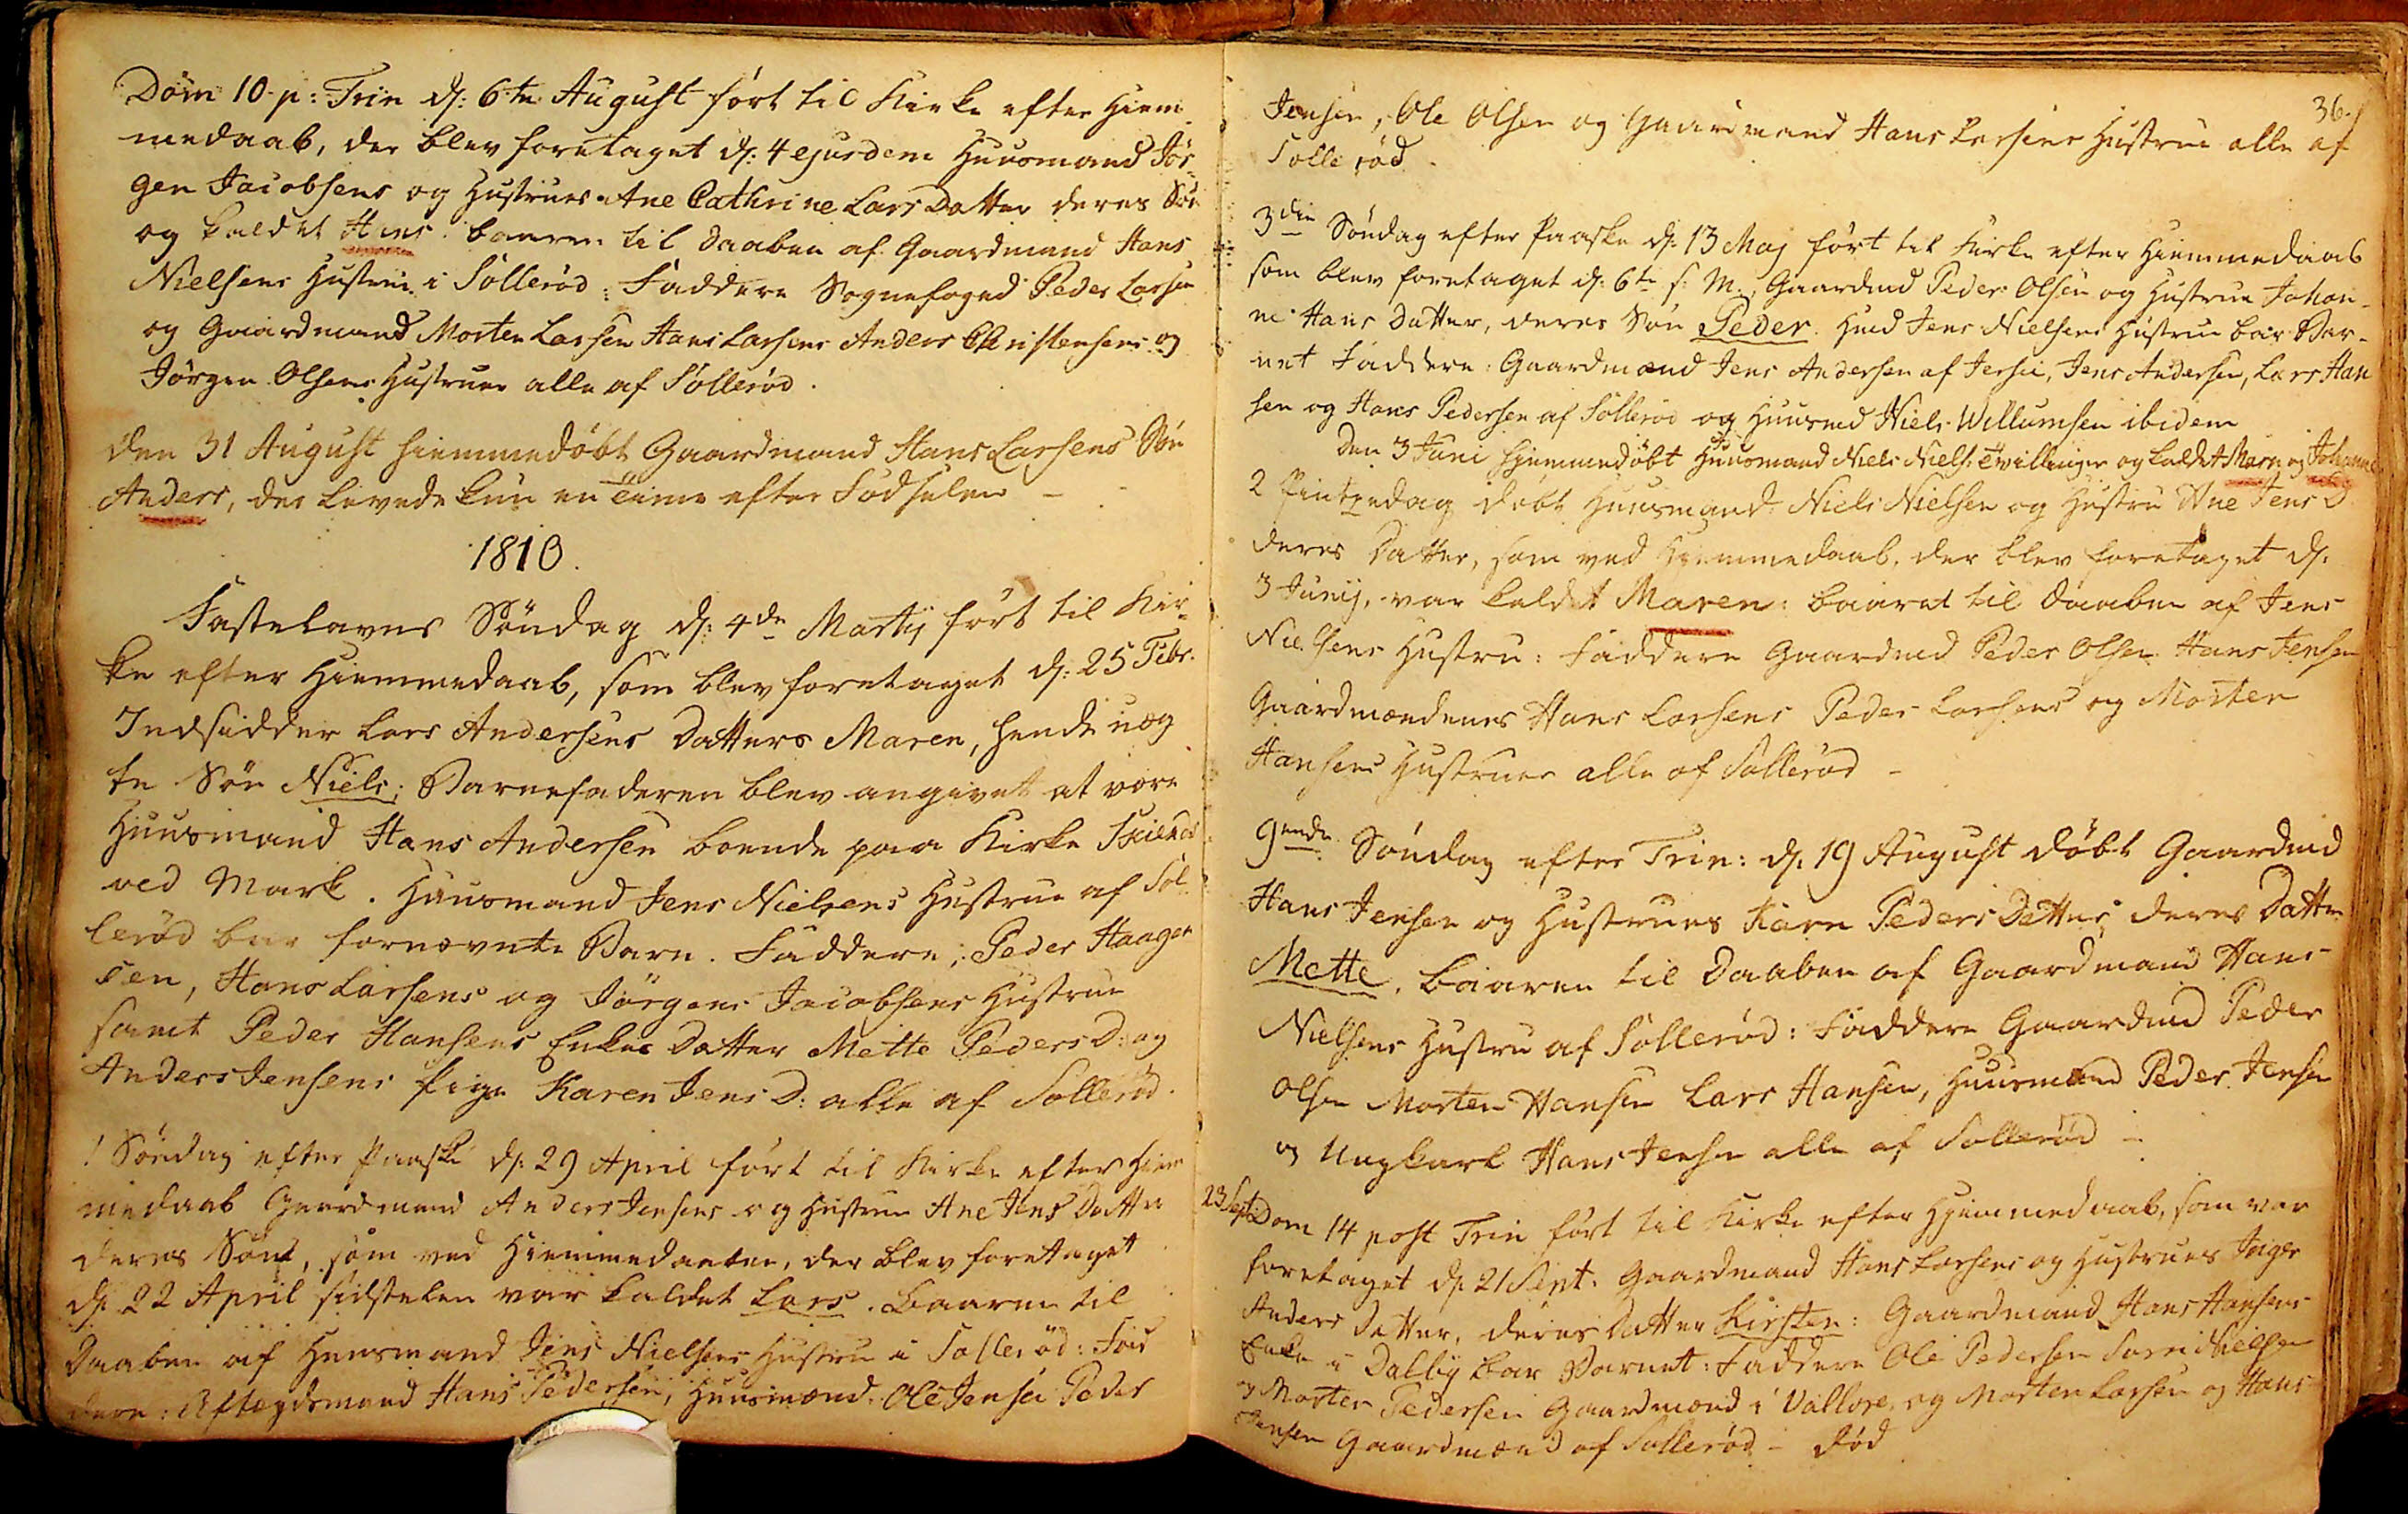Dom: 10 p: Trin d: 6te August ført til Kirke efter Hiem
medaab, der blev foretaget d: 4 ejusdem Huusmand Jør¬
gen Jacobsens og Hustrues Ane Cathrine Lars Datter deres Søn
og kaldet Hans. baaren til Daaben af Gaardmand Hans
Nielsens Hustrue i Sollerød:Faddere Sognefoged Peder Larsen
og Gaardmænd Morten Larsens Hans Larsens Anders Christensens og
Jørgen Olsens Hustrue alle af Sollerød.
den 31 August hiemmedøbt Gaardmand Hans Larsens Søn
Anders, der levede kun en Time efter Fødselen
1810.
Fastelavns Søndag d: 4de Martij ført til Kir¬
ke efter Hiemmedaab, som blev foretaget d: 25 Febr.
Indsidder Lars Andersens Datters Maren, hendes uæg
te Søn Niels; Barnefaderen blev angivet at være
Huusmand Hans Andersen boende paa Kirke Skiends
ved Mark. Huusmand Jens Nielsens Hustrue af Sol
lerød bar fornævnte Barn. Faddere; Peder Haagen
sen, Hans Larsens og Jørgen Jacobsens Hustrue
samt Peder Hansens Enkes Datter Mette Peders D: og
Anders Jensens Pige Karen Jens D: alle af Sollerød.
1 Søndag efter Paaske d: 29 April ført til Kirke efter Hiem
medaab Gaardmand Anders Jensens og Hustrue Ane Jens Datter
deres Søn, som ved Hiemmedaaben, der blev foretaget
d: 22 April sidstehen## var kaldet Lars. Baaren til
Daaben af Huusmand Jens Nielsens Hustrue i Sollerød: Fad
dere: Aftægtsmand Hans Pedersen, Huusmænd Ole Jensen Peder
36.
Jensen, Ole Olsen og Gaardmand Hans Larsens Hustrue alle af
Sollerød.
3die Søndag efter Paaske d: 13 Maj ført til Kirke efter Hiemmedaab
som blev foretaget d: 6te s: M:, Gaardmand Peder Olsen og Hustrue Johan¬
ne Hans Datter, deres Søn Peder. Hmd Jens Nielsens Hustrue bar Bar¬
net Faddere: Gaardmænd Jens Andersen af Jersie, Jens Andersen, Lars Han
sen og Hans Pedersen af Sollerød og Huusmd Niels Willumsen ibidem
Den 3 Juni Hiemmedøbt Huusmand Niels Niels: Tvillinger og kaldet Marn og Johanne
2 Pintzedag døbt Huusmand Niels Nielsen og Hustru Ane Jens D:
deres Datter, som ved Hiemmedaab, der blev foretaget d:
3 Junij, var kaldet Maren: baaret til Daaben af Jens
Nielsens Hustrue: Faddere: Gaardmd Peder Olsen Hans Jensen
Gaardmændenes Hans Larsens Peder Larsens og Morten
Hansens Hustruer alle af Sollerød
9ende Søndag efter Trin: d: 19 August døbt Gaardmd
Hans Jensen og Hustrues Karn Peders Datter, deres Datter
Mette. baaren til Daaben af Gaardmand Hans
Nielsens Hustru af Sollerød: Faddere Gaardmd Peder
Olsen Morten Hansen Lars Hansen, Huusmand Peder Jensen
og Ungkarl Hans Jensen alle af Sollerød
23 Sept: Dom 14 post Trin ført til kirke efter Hjemmedaab, som var
foretaget d: 21 Sept: Gaardmand Hans Larsens og Hustrues Inger
Anders Datter, deres Datter Kirsten: Gaardmand Hans Hansens
Enke i Dalby bar Barnet: Faddere Ole Pedersen Sørn Nielsen
og Morten Pedersen Gaardmand i Vallore, og Morten Larsen og Hans
Jensen Gaardmænd af Sollerød - død